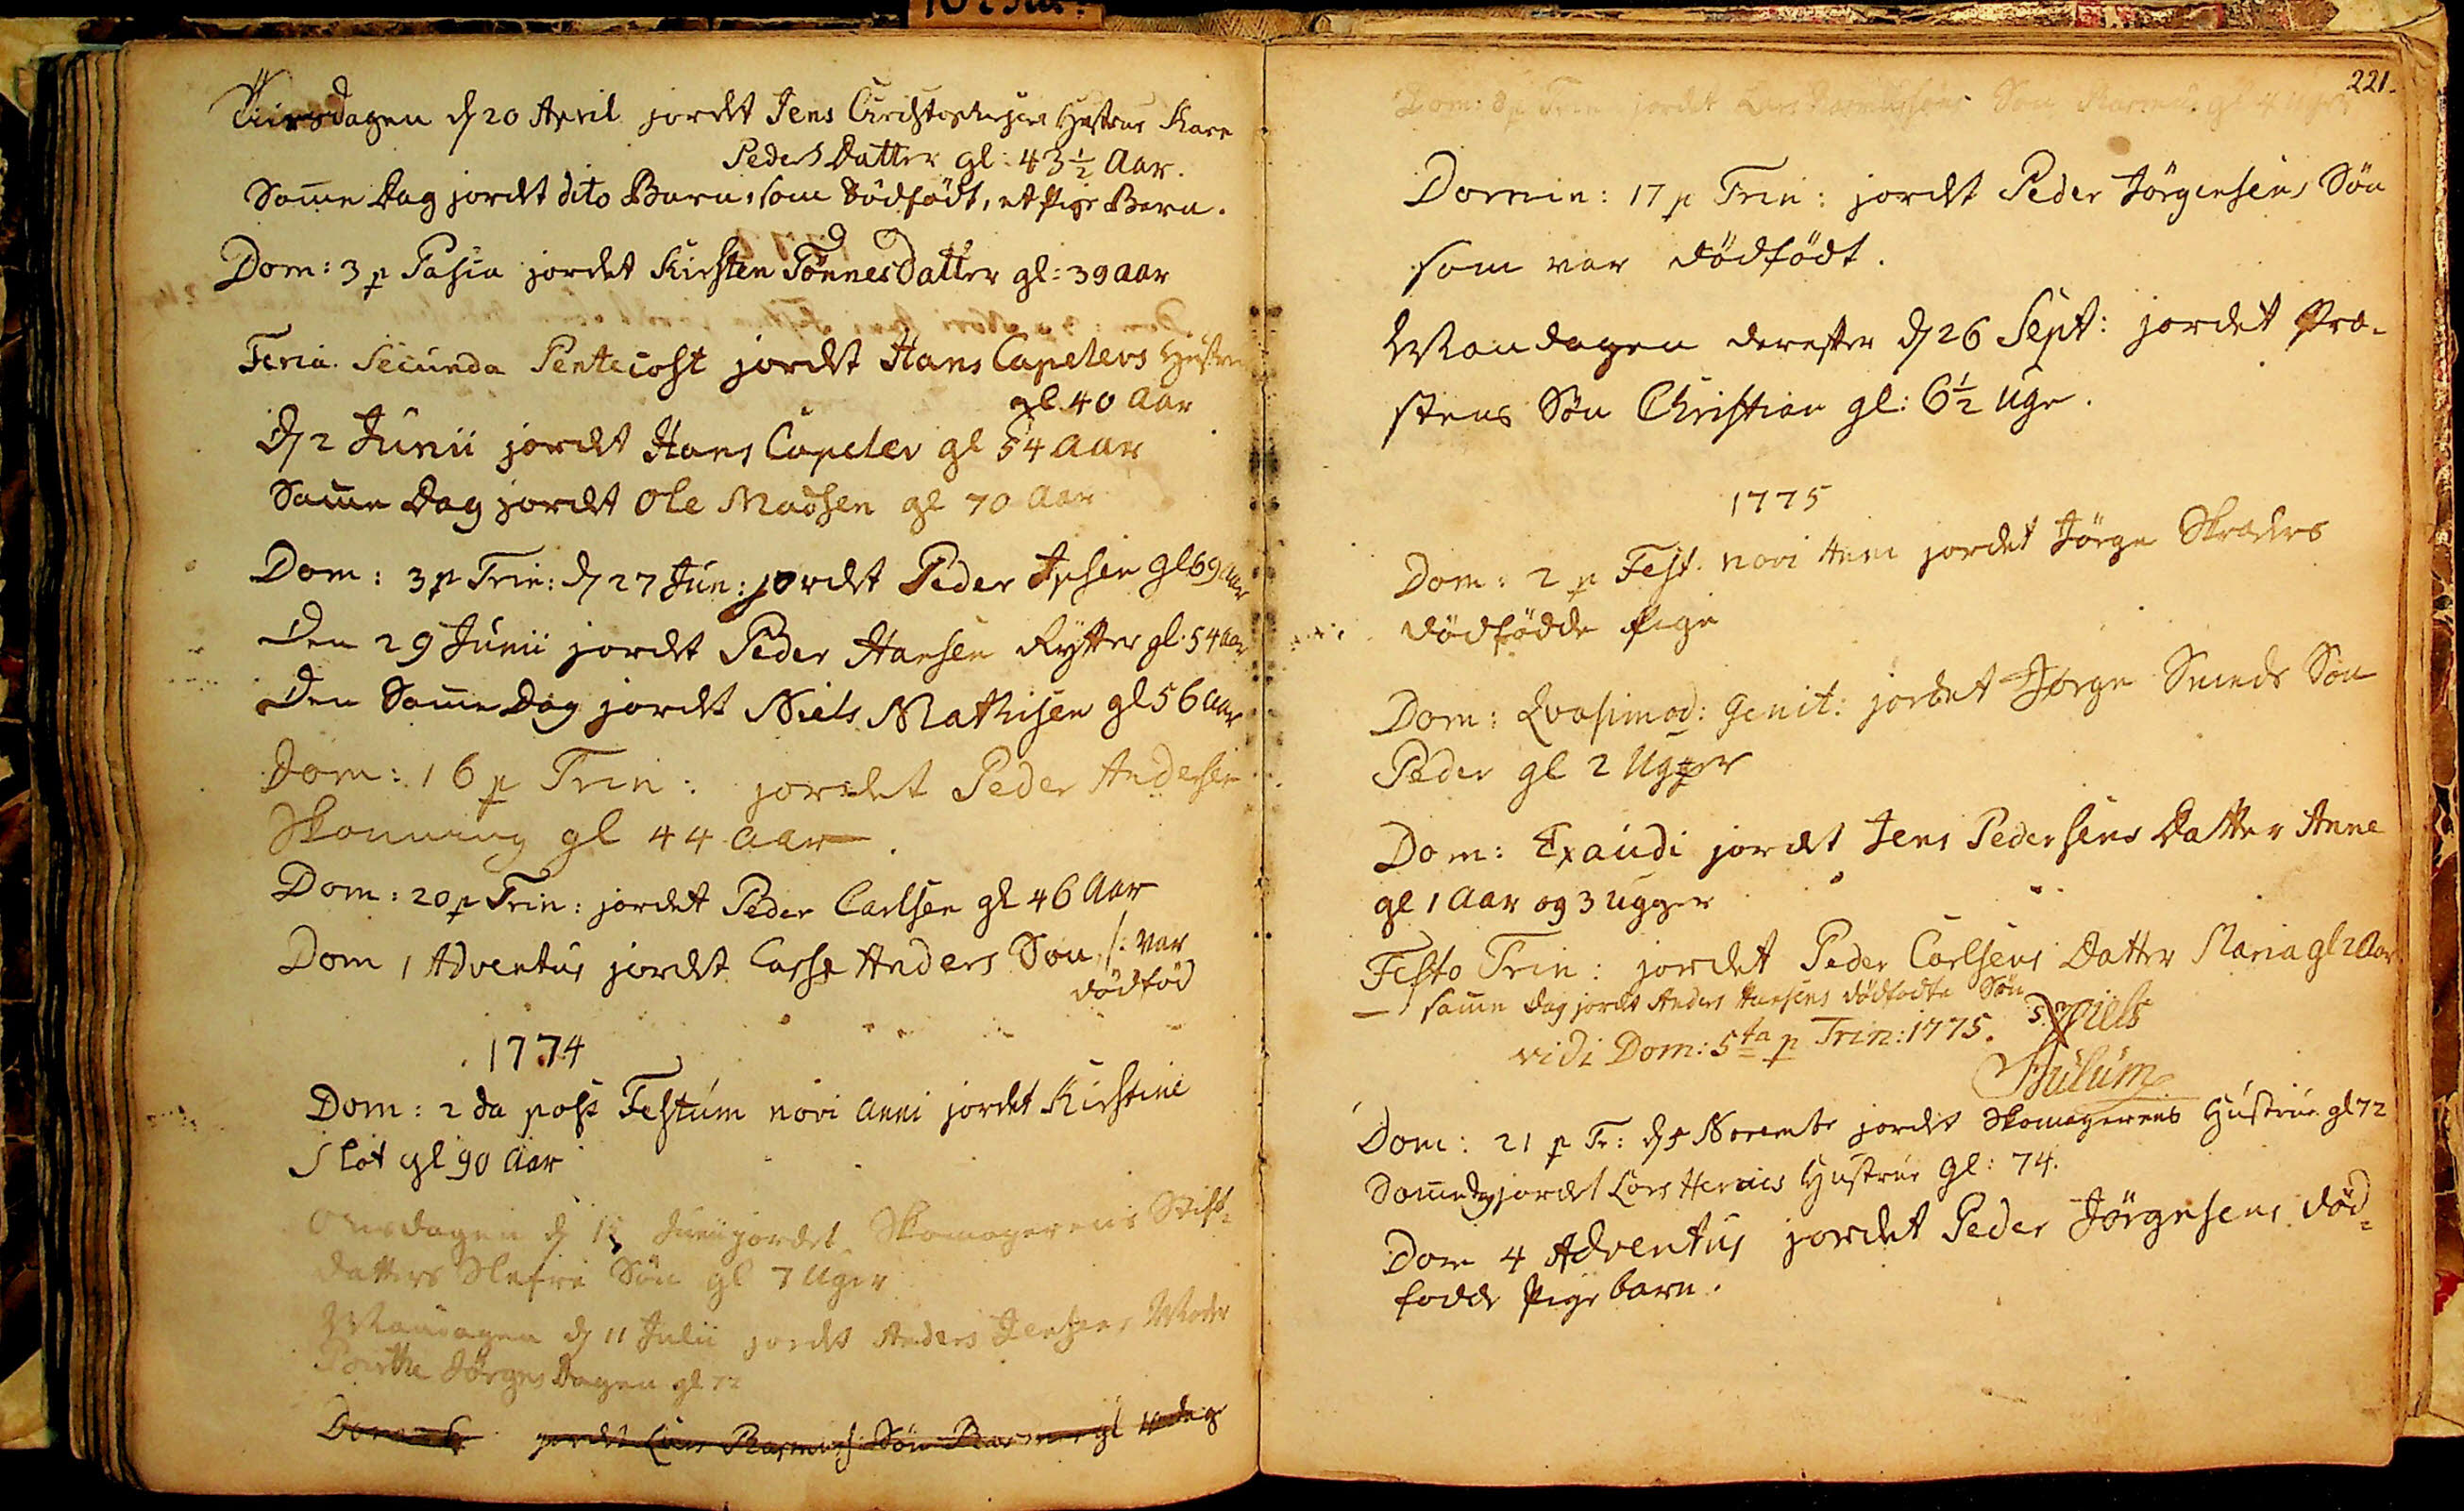Tiirsdagen d 20 April jordt Jens Christophersens Hustrue Karn
Peders Datter gl: 43½ Aar
Samme Dag jordt dito Barn, som dødfødt, et Pige Barn.
Dom: 3 p Pasca jordet Kirsten Tønnesdatter gl: 39 Aar
Feria. Secunda Pentecost jordt Hans Capelevs Hustrue
gl 40 Aar
d 2 Junii jordet Hans Capelev gl 54 Aar
Samme Dag jordet Ole Madsen gl 70 Aar
Dom: 3 p Trin: d 27 Jun: jordt Peder Ipsen gl 69 Aar
Den 29 Junii jordt Peder Hansen Rytter gl. 54 Aar
Den Samme Dag jordet Niels Mathisen gl 56 Aar
Dom: 16 p Trin: jordet Peder Andersen
Skonning gl 44 Aar
Dom: 20 p Trin: jordet Peder Carlsen gl 46 Aar
Dom 1 Adventus jordet Carhs## Anders Søn /. var
dødfød
1774
Dom: 2da post Festum novi Anni jordet Kirstine
Slot gl 90 Aar
Onsdagen d 15 Junii jordet Skomagerens Stift¬
datters Slefri Søn gl 7 Uger
Mandagen d 11 Julii jordet Anders Jensens Moder
Birthe Jørgns. Dagen## gl 72
Dom ## jordet Lars Rasmus: Søn Rasmus gl 10 Dage
221.
Dom: 8 p Trin: jordet Lars Rasmussøns Søn Rasmus gl 4 Uger
Domin: 17 p Trin: jordt Peder Jørgensens Søn
som var dødfødt.
Mandagen derefter d 26 Sept: jordet Præ¬
stens Søn Christian gl: 6½ Uge
1775
Dom: 2 p Fest: novi Anni jordet Jørgn Skræders
dødfødde Pige
Dom: Qvasimod: Genit: jordet Jørgn Smeds Søn
Peder gl 2 Ugger
Dom: Exaudi jordt Jens Pedersens Datter Anne
gl 1 Aar og 3 Ugger
Festo Trin: jordet Peder Carlsens Datter Maria gl 2 Aar
- samme Dag jordt Anders Hansens dødfødte Søn
Vidi Dom: 5ta p Trin: 1775. Sgr Niels
Aulum
Dom: 21 p Tr: d 5 Novembr jordt Skomagerens Hustrue gl 72
Samme dag jordt Lars Henrics Hustrue gl: 74.
Dom 4 Adventus jordet Peder Jørgnsens død¬
fødde Pigebarn.
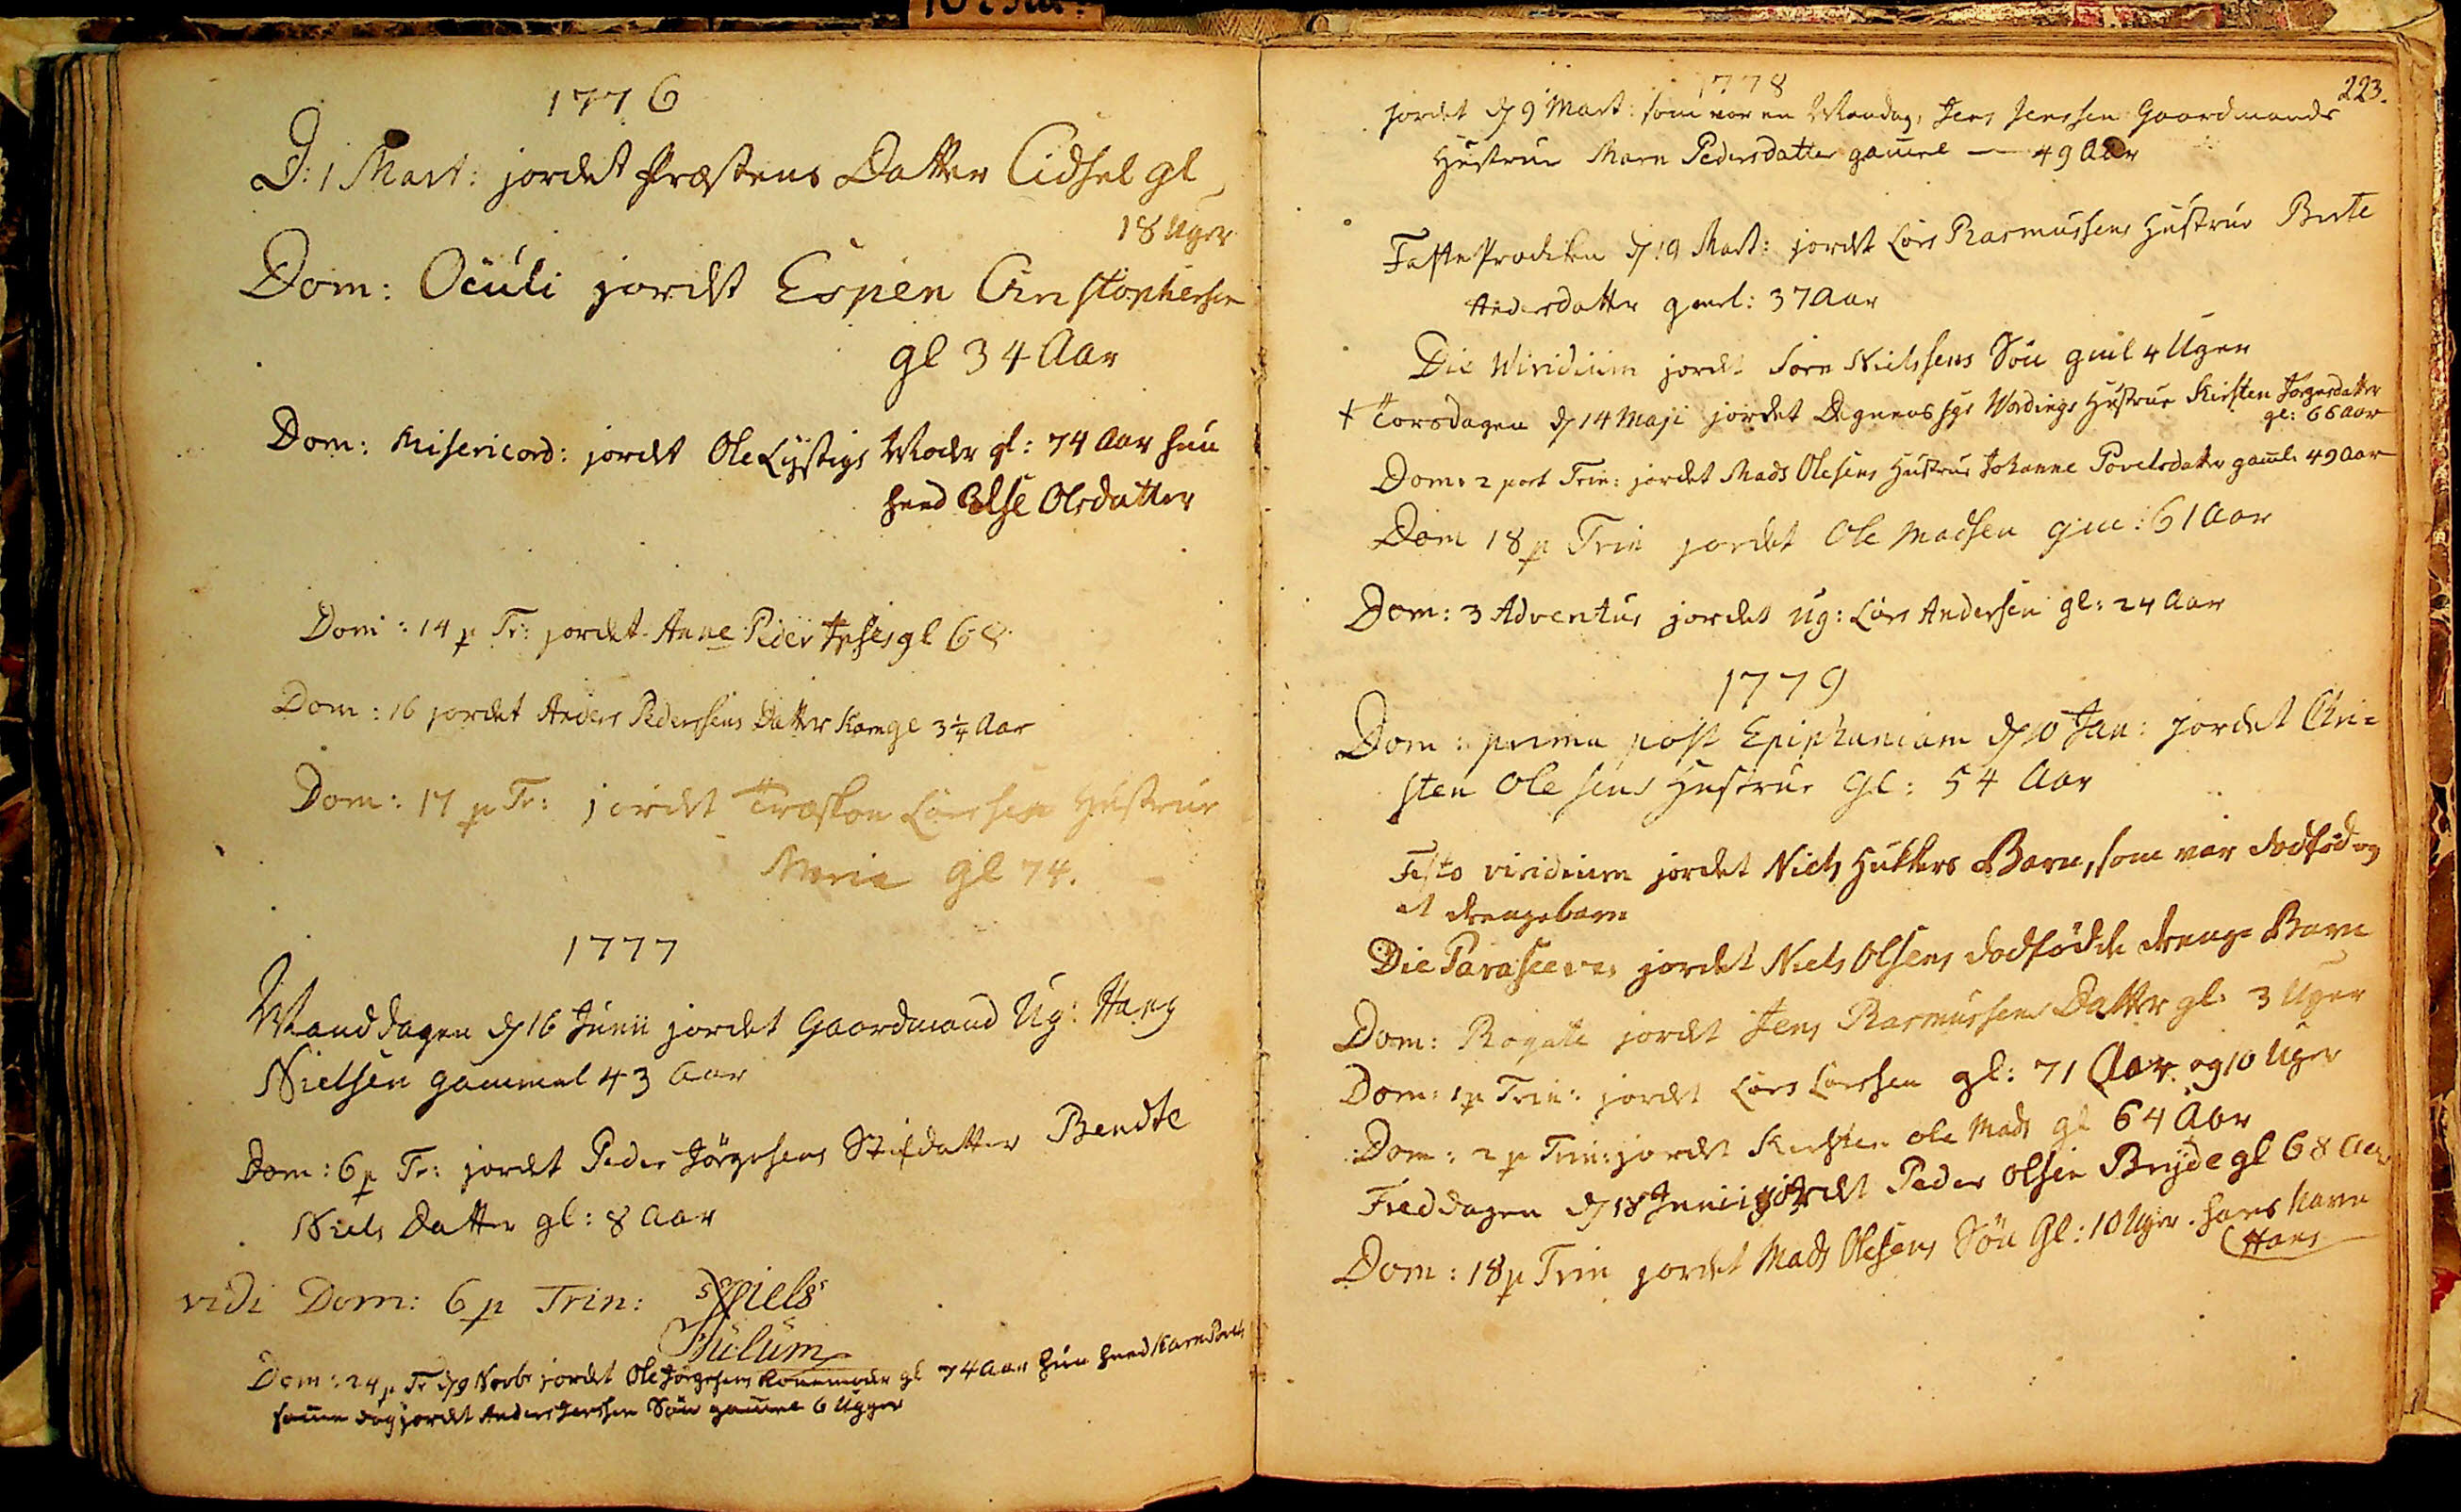1776
D: 1 Mart: jordet Præstens Datter Cidsel gl 
18 Uger
Dom: Oculi jordt Espen Christophersen
gl 34 Aar
dom: Misericord: jordt Ole Lystigs Modr gl: 74 Aar hun
heed Else Olsdatter
Dom: 14 p Tr: jordet: Anne Peder Ipses gl 68
Dom: 16 jordet Anders Pedersens Datter Karn gl 3¼ Aar
Dom: 17 p Tr: jordt Træskoe Larsis Hustrue
Marie gl 74.
1777
Mandagen d 16 Junii jordet Gaardmand Ug: Hans 
Nielsen gammel 43 Aar
Dom: 6 p Tr: jordt Peder Jørgnsens Stifdatter Bendte
Niels Datter gl: 8 Aar
Vidi Dom: 6 p Trin: 
Sgr Niels
Aulum
Dom: 24 p Tr d 9 Novbr jordt Ole Jørgnsens Konemoder gl 74 Aar hun heed Karn Povels
samme dag jordt Anders Jenssen Søn gammel 6 Ugger
223.
1778
Jordet d 9 Mart: som var en Mandag: Jens Jenssen Gaardmands
Hustrue Marn Pedersdatter gammel ___ 49 Aar
Fasteprædiken d 19 Mart: jordt Lars Rasmussens Hustrue Birte
Andersdatter gmel: 37 Aar
Die Wiridium jordt Sørn Nielssens Søn gml 4 Uger
Torsdagen d 14 Maji jordet Degnens Sgr Wordings Hustrue Kirsten Jørgnsdatter
gl: 66 Aar
Dom: 2 post Trin: jordet Mads Olesens Hustrue Johanne Povelsdatter gammel 49 Aar
Dom 18 p Trin jordet Ole Madsen gm: 61 Aar
Dom: 3 Adventus jordet Ug: Lars Andersen gl: 24 Aar
1779
Dom: prima post Epiphaniam d 10 Jan: jordet Chri¬
sten Olesens Hustrue gl: 54 Aar
Festo viridium jordet Niels Hukkers Barn, som var dødfød og 
et drengebarn
Die Parasceves jordet Niels Olsens dødfødde drenge Barn
Dom: Rogate jordt Jens Rasmussens Datter gl: 3 Uger
Dom: 1 p Trin: jordt Lars Larssen gl: 71 Aar og 10 Uger
Dom: 2 p Trin: jordt Kirsten Ole Mads gl 64 Aar
Freddagen d 18 Junii jordt Peder Olsen Bryde gl 68 Aar
Dom: 18 p Trin jordet Mads Olesens Søn  gl: 10 Uger. hans Navn 
Hans.